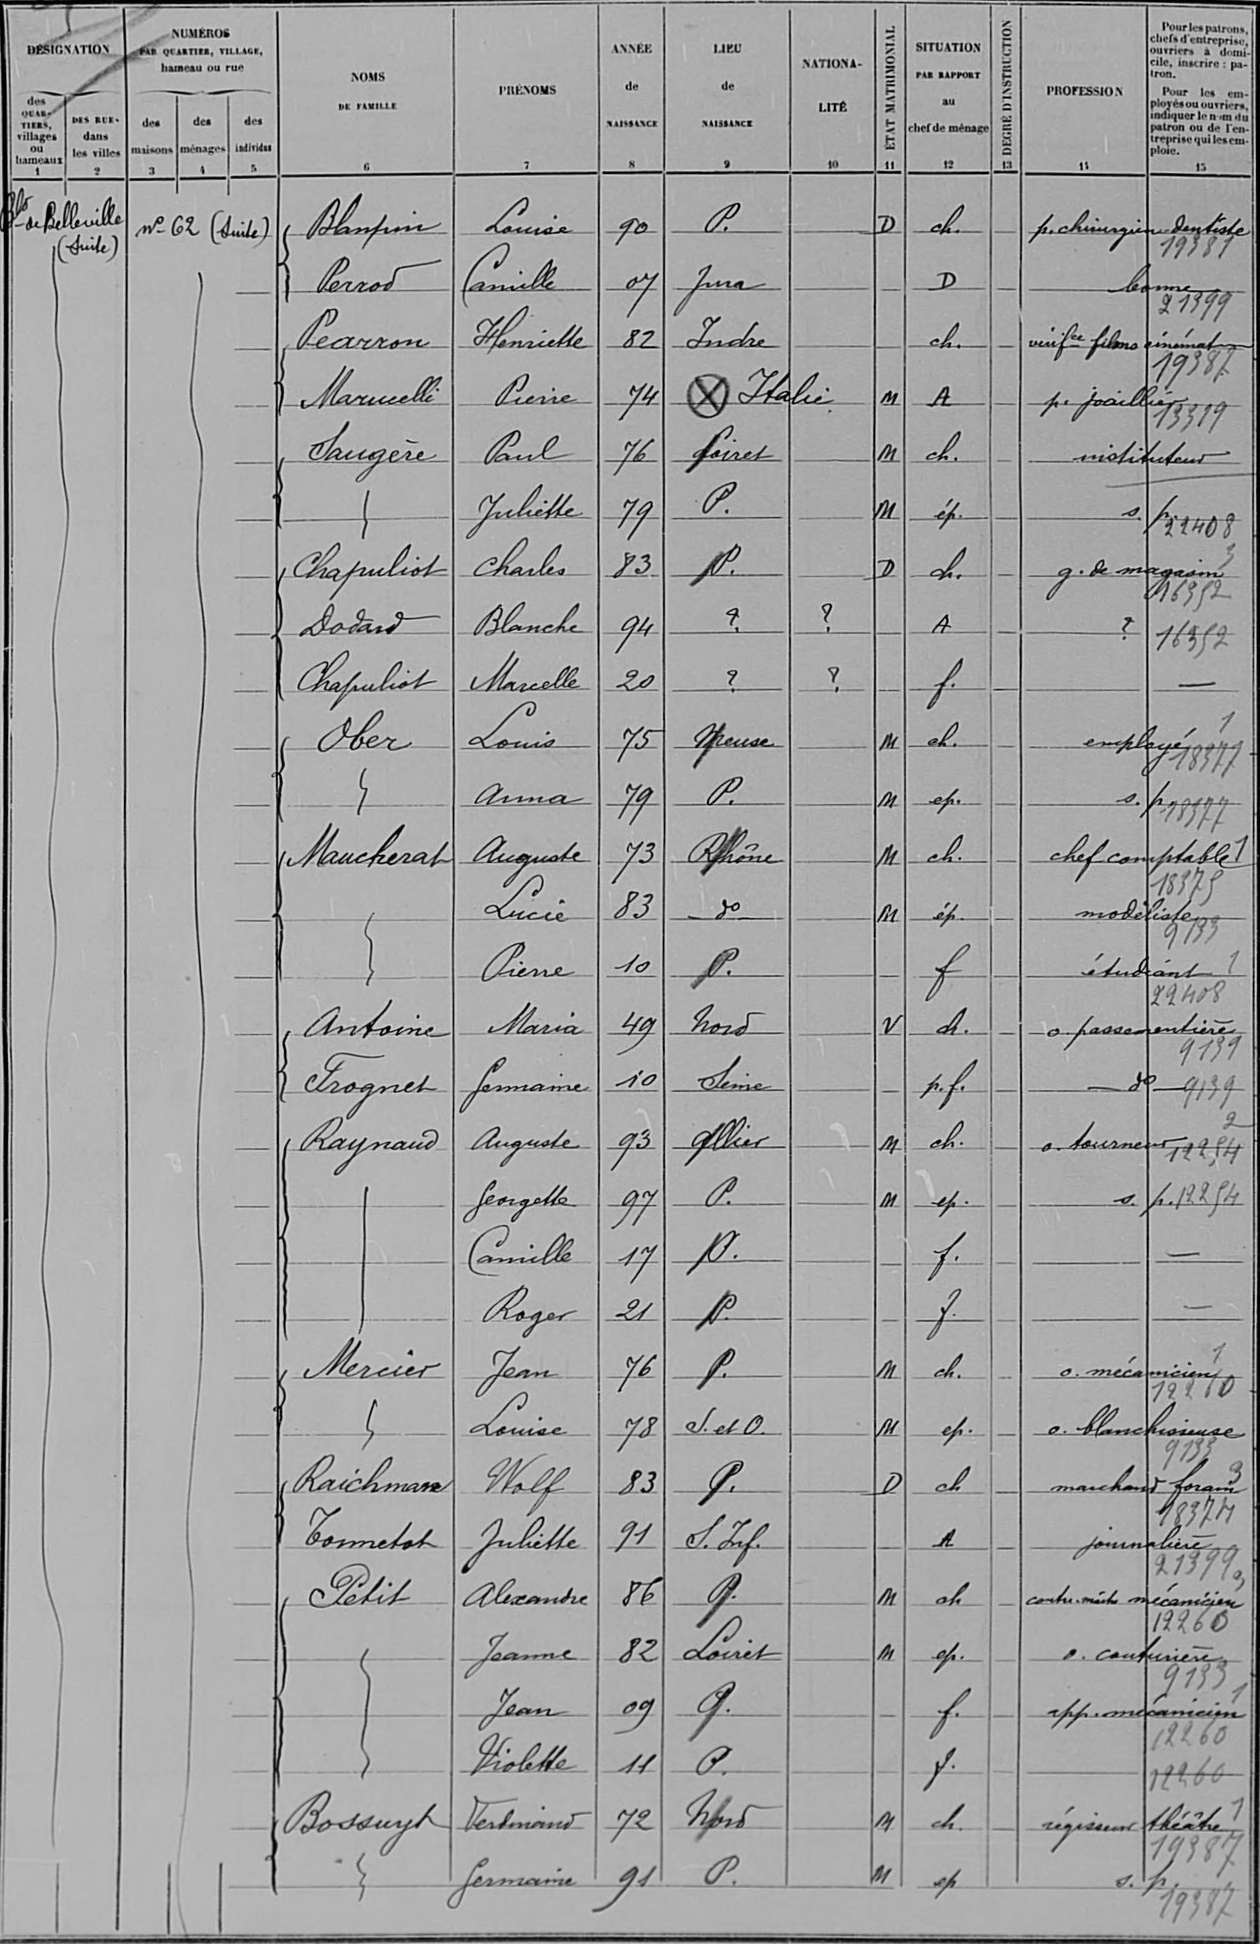Blanpin/Louise/90/P /¤/D/ch /¤/p chirurgien dentiste/¤
Perrod/Camille/07/Jura/¤/¤/D/¤/bonne/¤
Pearron/Henriette/82/Indre/¤/¤/ch /¤/vérif films cinémat/¤
Marucelli/Pierre/74/Italie/¤/M/A/¤/p joaillier/¤
Saugère/Paul/76/Loiret/¤/M/ch /¤/instituteur/¤/¤
¤/Juliette/79/P /¤/M/ép /¤/s p /22408
Chapuliot/Charles/83/P /¤/D/ch /¤/g de magasin/¤
Dodard/Blanche/94/¤/¤/¤/A/¤/¤/16352
Chapuliot/Marcelle/20/¤/¤/¤/f /¤/¤/¤/¤
Ober/Louis/75/Meuse/¤/M/ch /¤/employé/¤
¤/Anna/79/P /¤/M/ép /¤/s p /18377
Maucherat/Auguste/73/Rhône/¤/M/ch /¤/chef comptable/¤
¤/Lucie/83/d°/¤/M/ép /¤/modeliste/¤
¤/Pierre/10/P /¤/¤/f/¤/étudiant/¤
Antoine/Maria/49/Nord/¤/V/ch /¤/o passementière/¤
Frognet/Germaine/10/Seine/¤/¤/p f /¤/d°/9139 2
Raynaud/Auguste/93/Allier/¤/M/ch /¤/o tourneur/12254 2
¤/Georgette/97/P /¤/M/ep /¤/s p /12254
¤/Camille/17/P /¤/¤/f /¤/¤/¤/¤
¤/Roger/21/P /¤/¤/f /¤/¤/¤/¤
Mercier/Jean/76/P /¤/M/ch /¤/o mécanicien/¤
¤/Louise/78/S et O /¤/M/ep /¤/o blanchisseuse/¤
Raichman/Wolf/83/P /¤/D/ch/¤/marchand forain/¤
Tormetot/Juliette/91/S Inf /¤/¤/A/¤/journalière/¤
Petit/Alexandre/86/P /¤/M/ch/¤/contre-maître mécanicien/¤
¤/Jeanne/82/Loiret/¤/M/ep /¤/o couturière/¤
¤/Jean/09/P /¤/¤/f /¤/app mécanicien/¤
¤/Violette/11/P /¤/¤/f /¤/¤/12260
Bossuyt/Ferdinand/72/Nord/¤/M/ch /¤/régisseur théâtre/¤
¤/Germaine/91/P /¤/M/ep/¤/s p /19387
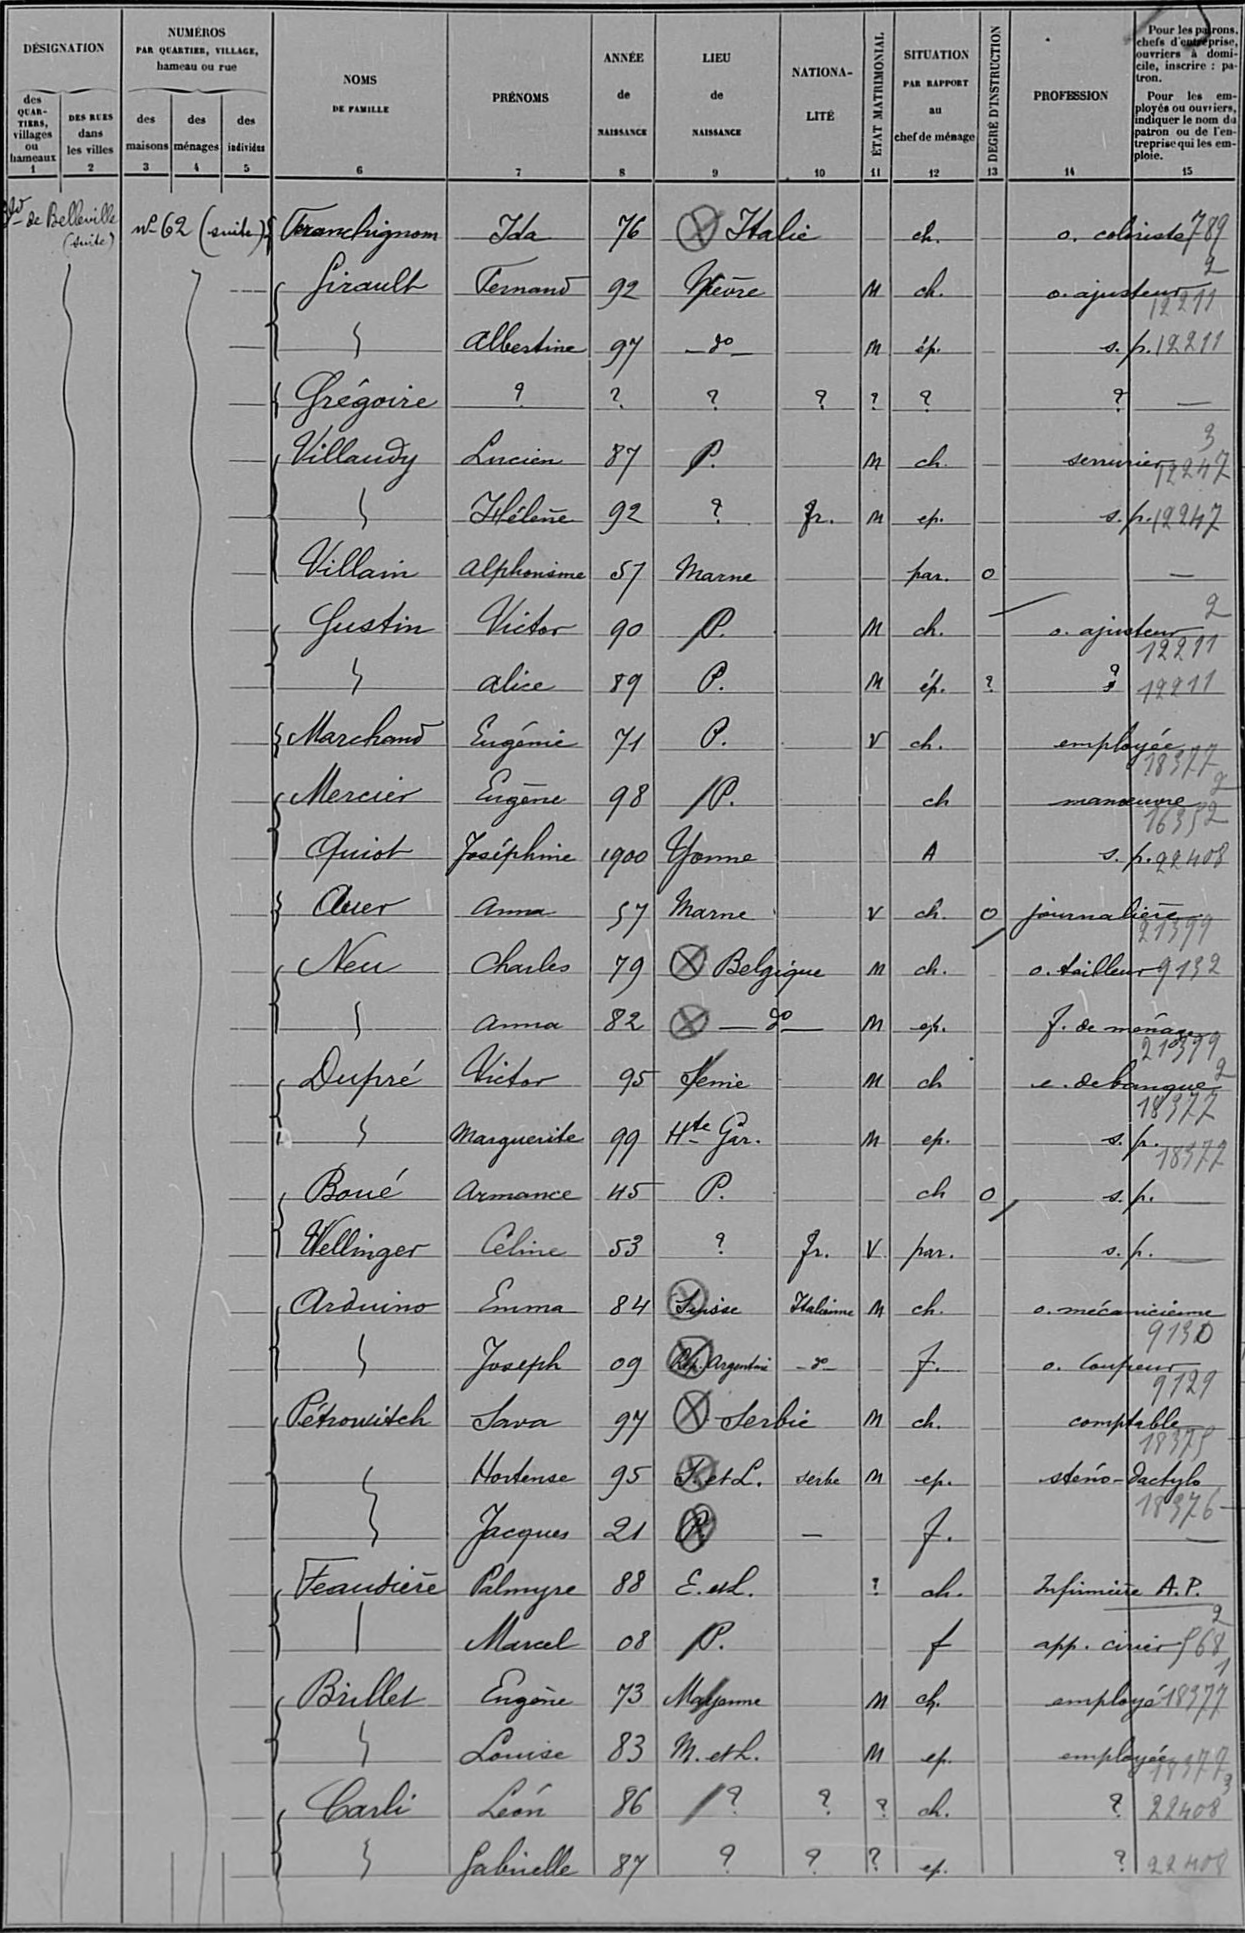Franchignon/Ida/76/Italie/¤/¤/ch /¤/o coloriste/789
Girault/Fernand/92/Nièvre/¤/M/ch /¤/o ajusteur/¤
¤/Albertine/97/d°/¤/M/ép /¤/s p /12211
Grégoire/¤/¤/¤/¤/¤/¤/¤/¤/¤
Villaudry/Lucien/87/P /¤/M/ch/¤/serrurier/12247
¤/Hélène/92/¤/fr /M/ep /¤/s p /12247
Villain/Alphonsine/57/Marne/¤/¤/par 0/¤/¤
Gustin/Victor/90/P /¤/M/ch /¤/o ajusteur/¤
¤/Alice/89/P /¤/M/ép /¤/¤/12211
Marchand/Eugène/71/P /¤/V/ch /¤/employée/¤
Mercier/Eugène/98/P /¤/¤/ch/¤/manoeuvre/¤
Quist/Joséphine/1900/Yonne/¤/¤/A/¤/s p /22408
Auer/Anna/57/MArne/¤/v/ch /0/journalière/¤
Neu/Charles/79/Belgique/¤/M/ch /¤/o tailleur/9132
¤/Anna/82/d°/¤/M/ep /¤/f de ménage/21399
Dupré/Victor/95/Seine/¤/M/ch/¤/e de banque/¤
¤/Marguertie/99/Hte Gar/¤/M/ep /¤/s p /¤
Boué/Armance/45/P /¤/¤/ch/0/s p /¤
Wellinger/Céline/53/¤/fr /V/par /¤/s p /¤
Arduino/Emma/84/Suisse/italienne/M/ch /¤/o mécanicienne/¤
¤/Joseph/09/Rep Argentine/d°/¤/f /¤/o coupeur/¤
Pétrowitsch/Sava/97/Serbie/¤/M/ch /¤/comptable/¤
¤/Hortense/95/S et L /Serbe/M/ep /¤/sténo-dactylo/¤
¤/Jacques/21/P /¤/¤/f /¤/¤/¤
Feaudière/Palmyre/88/E et L /¤/¤/ch /¤/Infirmère A P
¤/MArcel/09/P /¤/¤/f/¤/app cirier/568
Brillet/Eugène/73/Mayenne/¤/M/ch /¤/employé/18377
¤/Louise/83/M/et L /¤/M/ep /¤/employée/18377
Carli/Léon/86/¤/¤/¤/ch /¤/¤/22408
¤/Gabrielle/87/¤/¤/¤/ep /¤/¤/22408
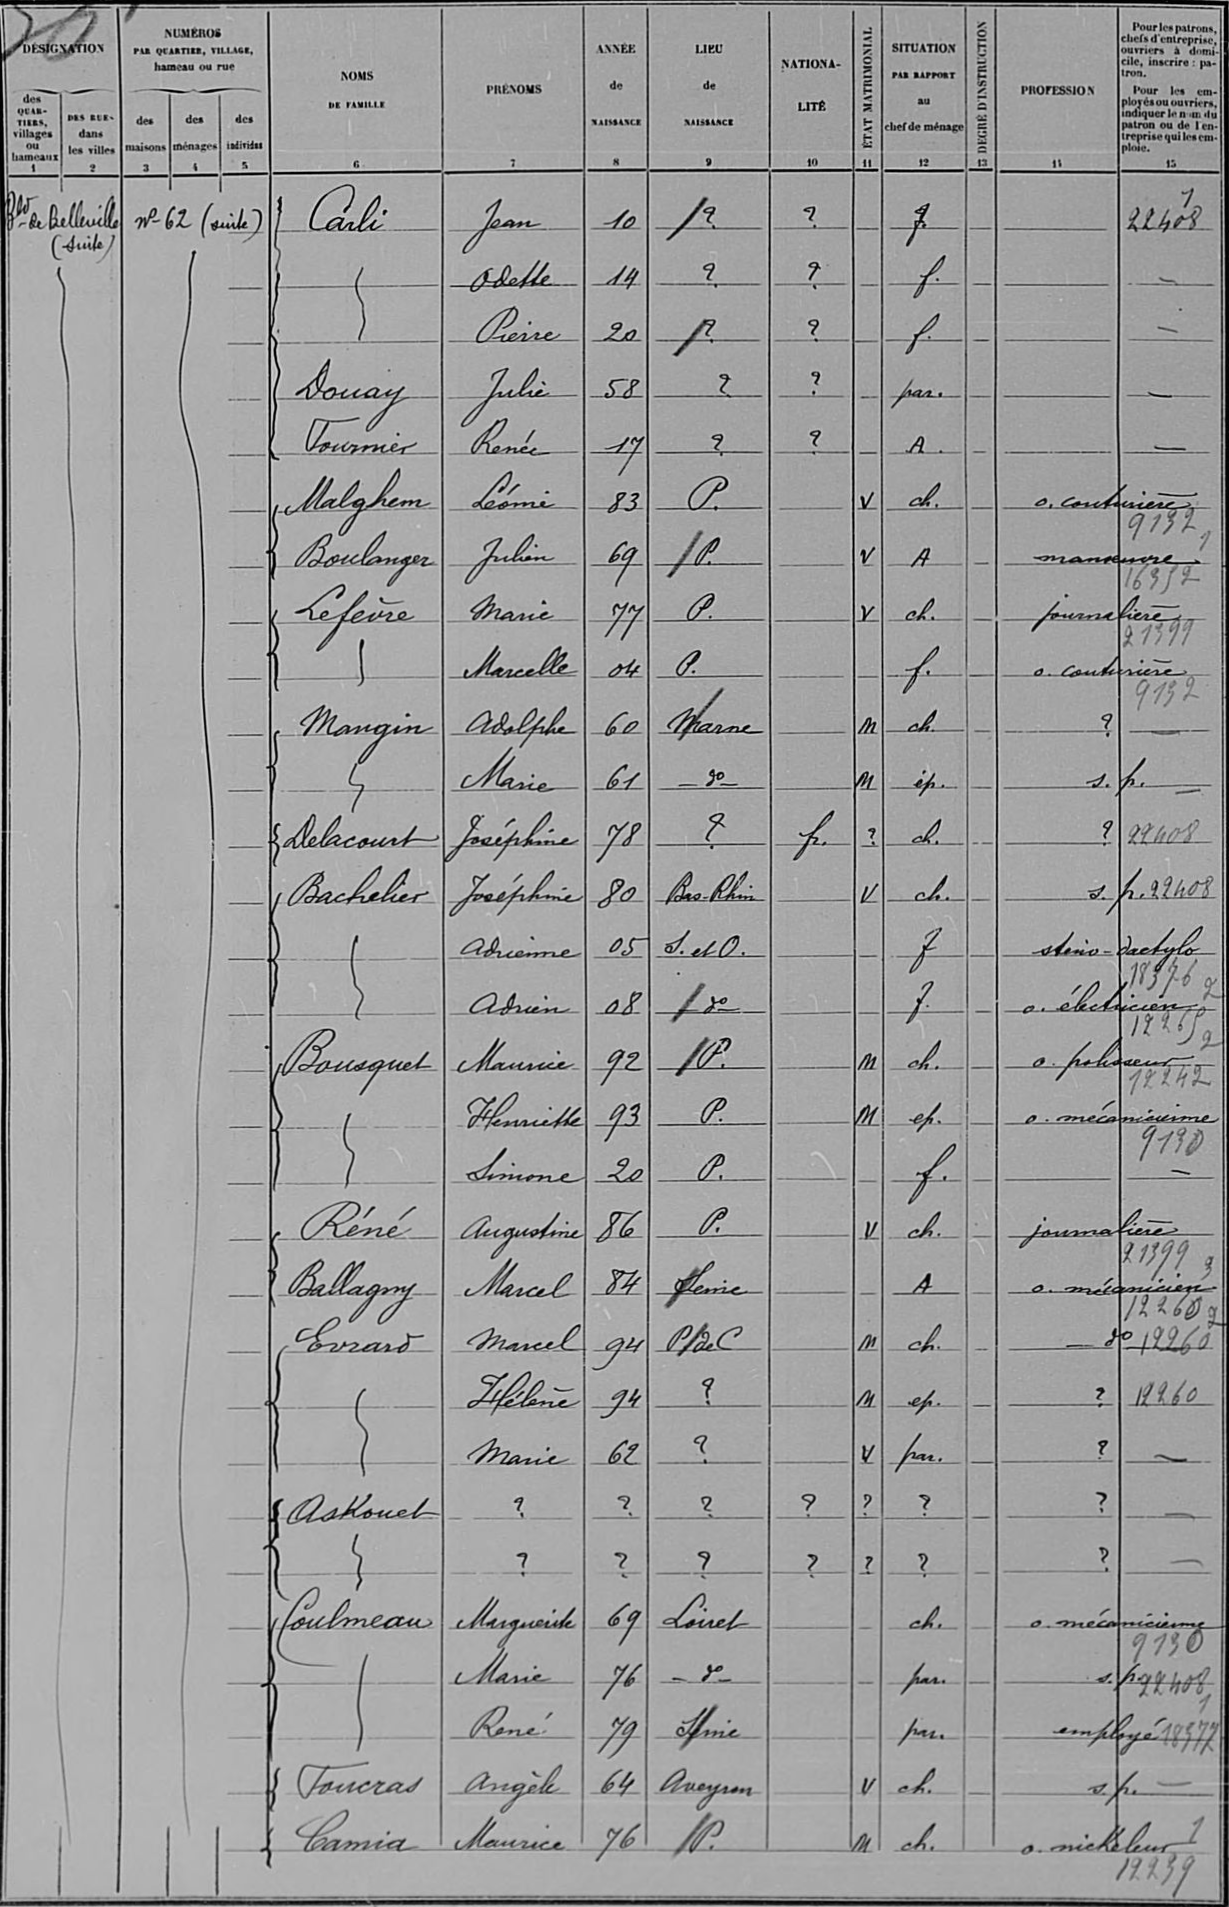Carli/Jean/10/¤/¤/¤/f/¤/¤/22408
¤/Odette/14/¤/¤/¤/f /¤/¤/¤
¤/Pierre/20/¤/¤/¤/f /¤/¤/¤
Douay/Julie/58/¤/¤/¤/par /¤/¤/¤
Fournier/Renée/17/¤/¤/¤/A/¤/¤/¤
Malghem/Léonie/83/P /¤/V/ch /¤/o coutuière/¤
Boulanger/Julien/69/P /¤/V/A/¤/manoeuvre/¤
Lefèvre/Marie/77/P /¤/V/ch /¤/journalière/¤
¤/MArcelle/04/P /¤/¤/f /¤/o couturière/¤
MAngin/Adolphe/60/Marne/¤/M/ch/¤/¤/¤
¤/MArie/61/d°/¤/M/ép /¤/s p /¤
Delacourt/Joséphine/78/¤/fr /¤/ch /¤/¤/22408
Bachelier/80/Bas Rhin/¤/V/ch /¤/s p /22408
¤/Adrienne/05/S et O /¤/¤/f/¤/sténo-dactylo/¤
¤/Adrien/08/d°/¤/¤/f /¤/o électricien/¤
Bousquet/Maurice/92/P /¤/M/ch /¤/o polisseur/¤
¤/Henriette/93/P /¤/M/ep /¤/o mécanicienne/¤
¤/Simone/20/P /¤/¤/f /¤/¤/¤
René/Augustine/86/P /¤/V/ch /¤/journalière/¤
Ballagny/MArcel/84/Seine/¤/¤/A/¤/o mécanicien/¤
Evrard/MArcel/94/P de C/¤/M/ch /¤/d°/12260
¤/Hélène/94/¤/¤/M/ep /¤/¤/12260
¤/MArie/62/¤/¤/V/par /¤/¤/¤
Ashouet/¤/¤/¤/¤/¤/¤/¤/¤/¤
¤/¤/¤/¤/¤/¤/¤/¤/¤/¤
Coulmeau/MArguerite/69/loiret/¤/¤/ch /¤/o mécanicienne/¤
¤/MArie/76/d°/¤/¤/par /¤/s p /2408
¤/René/79/Seine/¤/¤/par /¤/employé/18377
Foucras/Angèle/64/Aveyron/¤/V/ch /¤/s p /¤
Camia/Maurice/76/P /¤/M/ch /¤/o nickeleur/¤